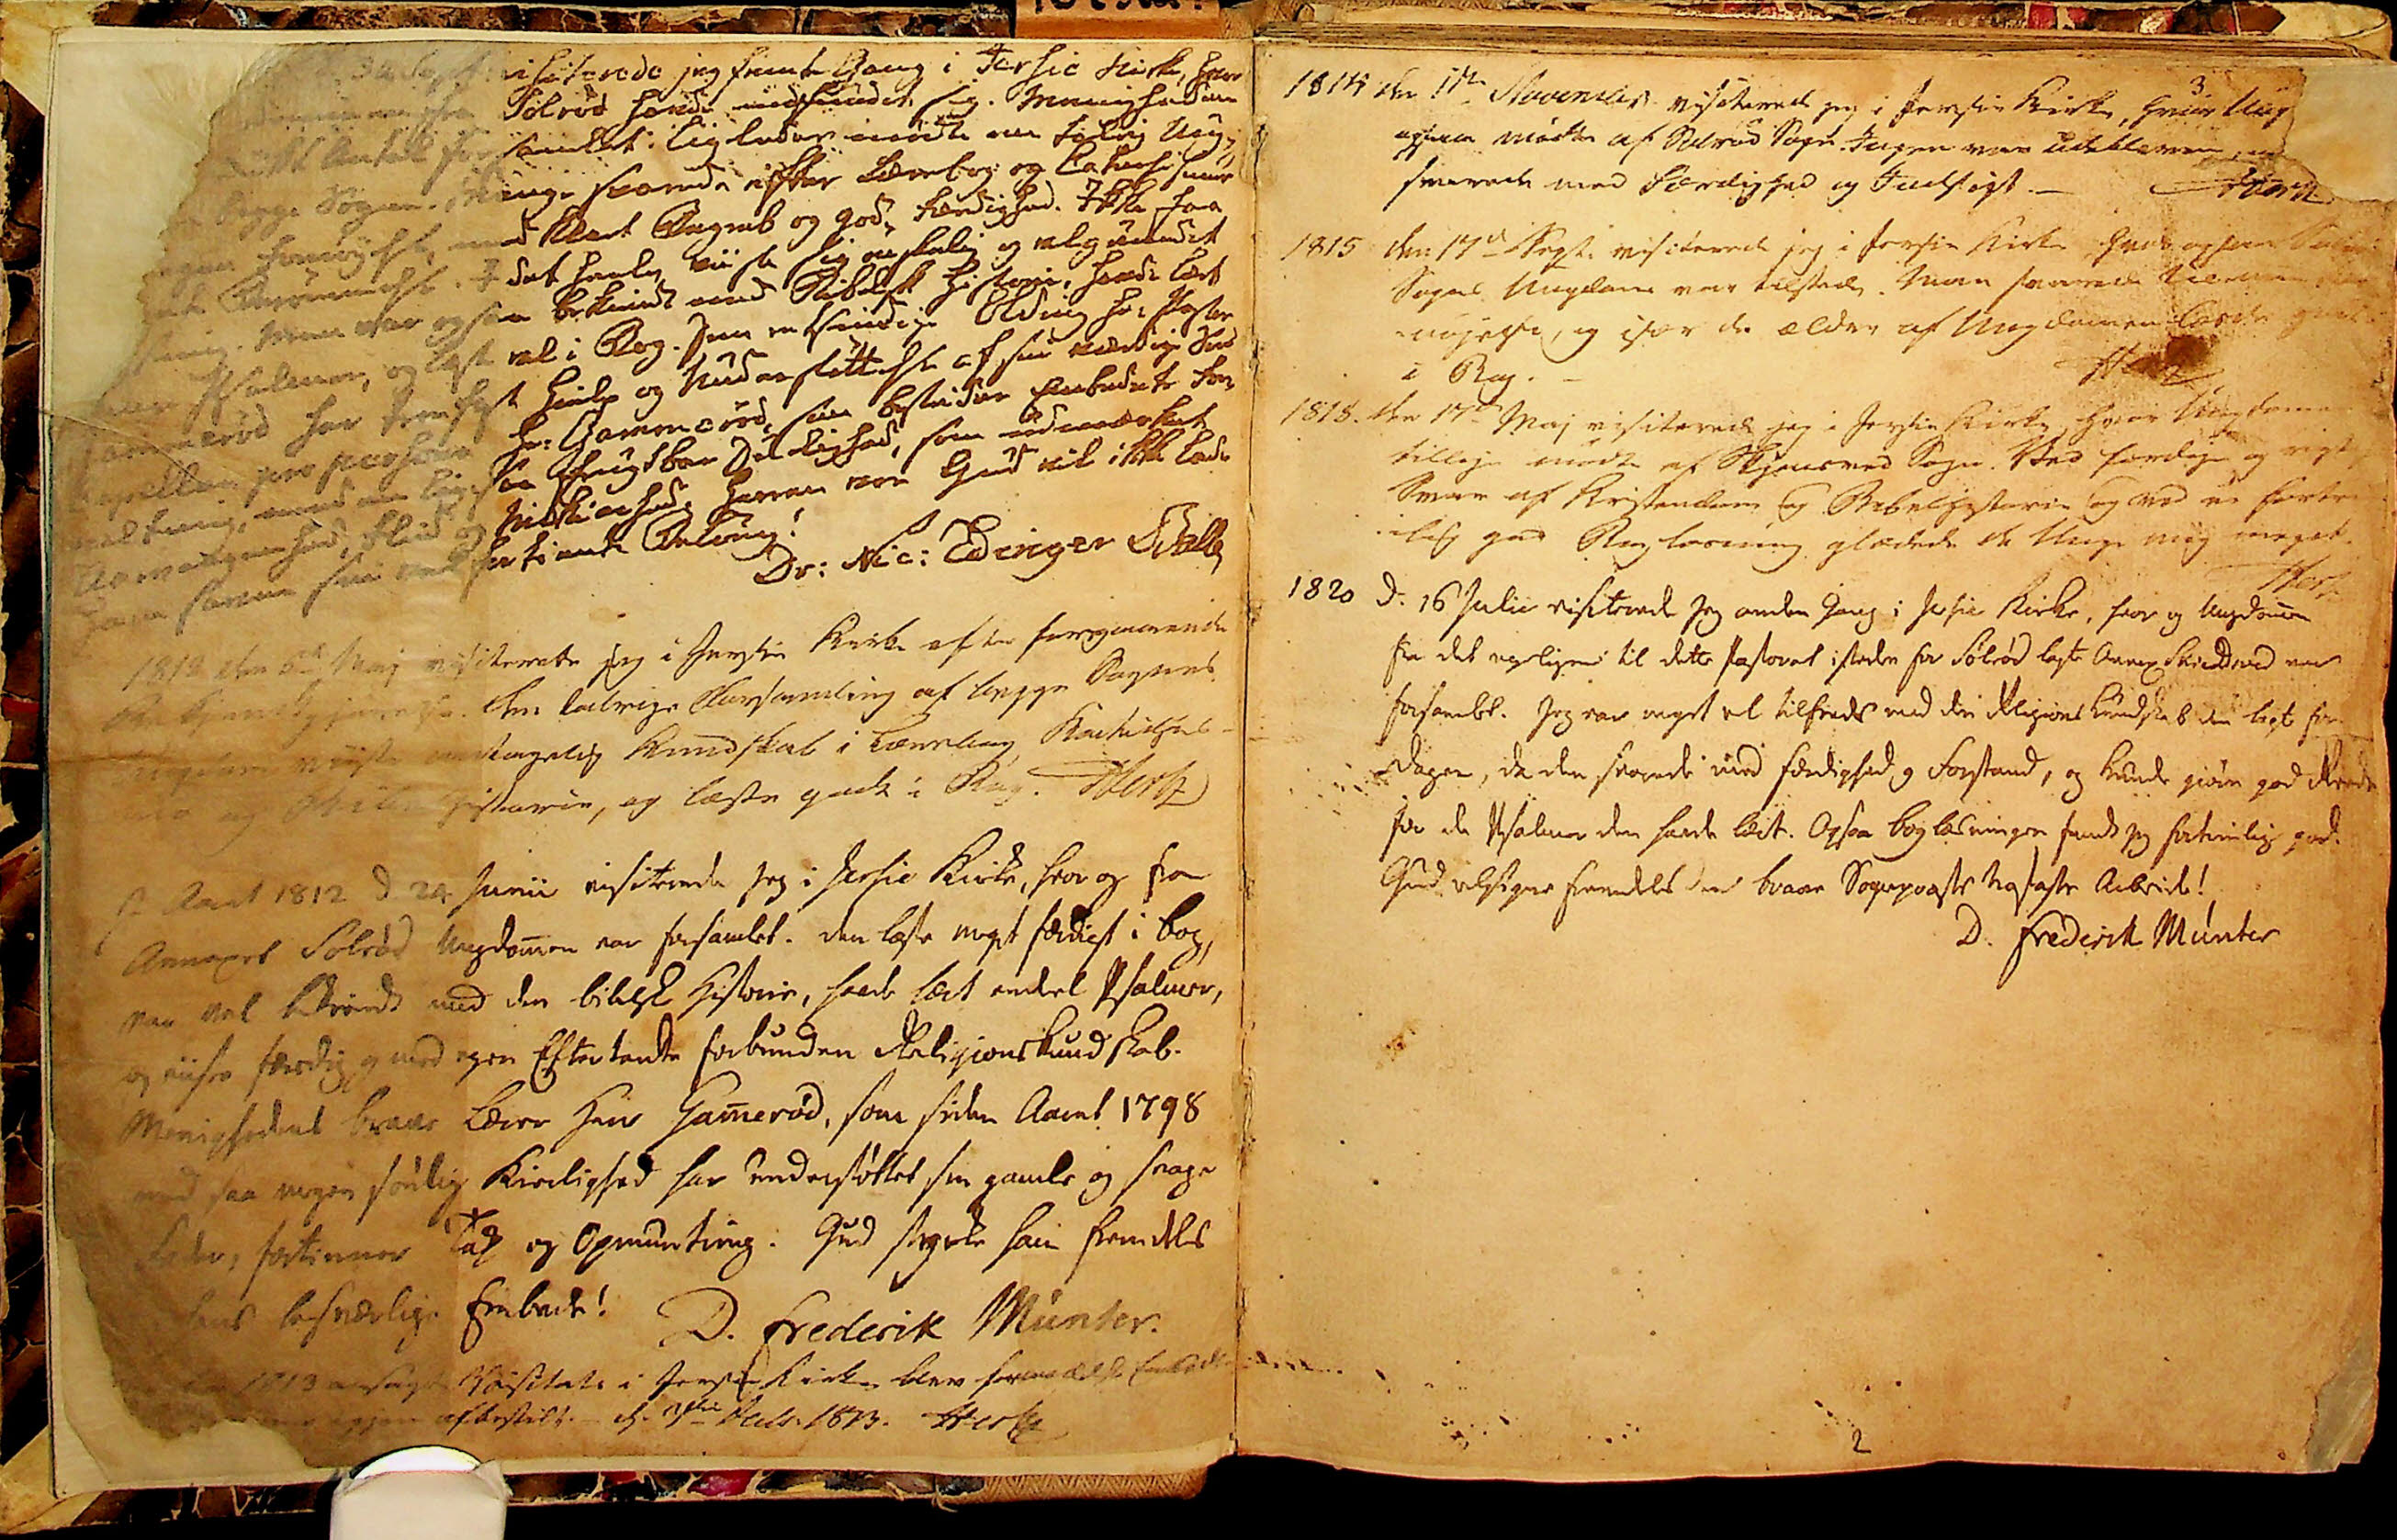30. Sept visiterede jeg femte Gang i Jersie Kirke, hvor
dommen fra Solrød havde indfundet sig. Menigheden
## Antal forsamlet. ligeledes mødte en herlig Ung¬
begge Sogne. Mange svarede efter Lærebog og Catechismus
egen fornøyelse , med klart Begreb og god færdighed. Ikke faa
## Berømmelse. I det heele viste sig ønskelig og velgrundet
sning. Man var ogsaa bekiendt med Bibelsk historie, havde lært
ange Psalmer, og læste vel i Bog. Som en Mündige Olding har Præsten
Gammerød her den faste Hiælp og Understøttelse af sin værdige Søn
Capellan pro persona Hr: Gammerød, som besider Embedets for¬
valtning, med en ligesaa frugtbar Duelighed, som udmærket
Aarvaagenhed, flid og Nidkierhed. Herren vor Gud vil ikke lade
ham savne sin velfortiente Beløning!
Dr: Nic: Edinger Balle
1812 den 5te Maj visiterede jeg i Jersie Kirke efter foregaaende
Bekjendtgjørelse. Den talrige Forsamling af begge Sognes
Ungdom viste antagelig Kundskab i Lærebog, Katechis¬
mus og Bibelsk historie, og læste godt i Bog.
Hertz
I Aaret 1812 d 24 Junii visiterede jeg i Jersie Kirke, hvor og fra
Annexet Solrød Ungdomen var forsamlet. den læste meget færdigt i Bog,
var vel bekiendt med den bibelske Historie, havde lært endel Psalmer,
og viiste færdig og med egen Eftertanke forbunden Religionskundskab.
Menighedens brave Lærer Herr Gammerød, som siden Aaret 1798
med saa megen sønlig Kierlighed har understøttet sin gamle og svage
Fader, fortiener Tak og Opmuntring. Gud styrke ham fremdeles
i hans besværlige Embede!
D. Friderik Münter
## 1813 ansagte ## Visitats i Jersie Kirke blev formedelst Embeds
igjen afbestilt. - d. 3die Decbr: 1813. Herts
3.
1814 den 1ste November visiterede jeg i Jersie Kirke, hvor Ung
ogsaa mødte af Solrød Sogn. Ingen var udeblevne, man
svarede med færdighed og Indsigt.
Hertz
1815 den: 17de Sept: visiterede jeg i Jersie Kirke, hvor ogsaa Solrød
Sogns Ungdom var tilstede. Man svarede til megen for¬
nøyelse, og især de ældre af ungdommen læsde godt
i Bog.
Hertz
1818 den 17de Maj visiterede jeg i Jersie Kirke, hvor Ungdommen
tillige mødte af Skjensved Sogn. Ved færdige og rigtige
Svar af Kristendom og Bibelhistorie og med en fortrin
lig god Boglæsning glædede de Unge mig meget
Hertz
1820 d. 16 Julii visiterede jeg anden Gang i Jersie Kirke, hvor og Ungdomen
fra det nyeligen til dette Pastorat i steden for Solrød lagte Annex Skiendsved var
forsamlet. Jeg var meget vel tilfreds ved den Religions Kundskab den lagte for
Dagen, da den svarede med færdighed og Forstand, og kunde giøre god Reede
for de Psalmer den havde læst. Ogsaa boglæsningen fandt jeg fortrinlig god.
Gud velsigne fremdeles den brave Sognepræsts højtagte Arbeide!
D D. Frederik Münter